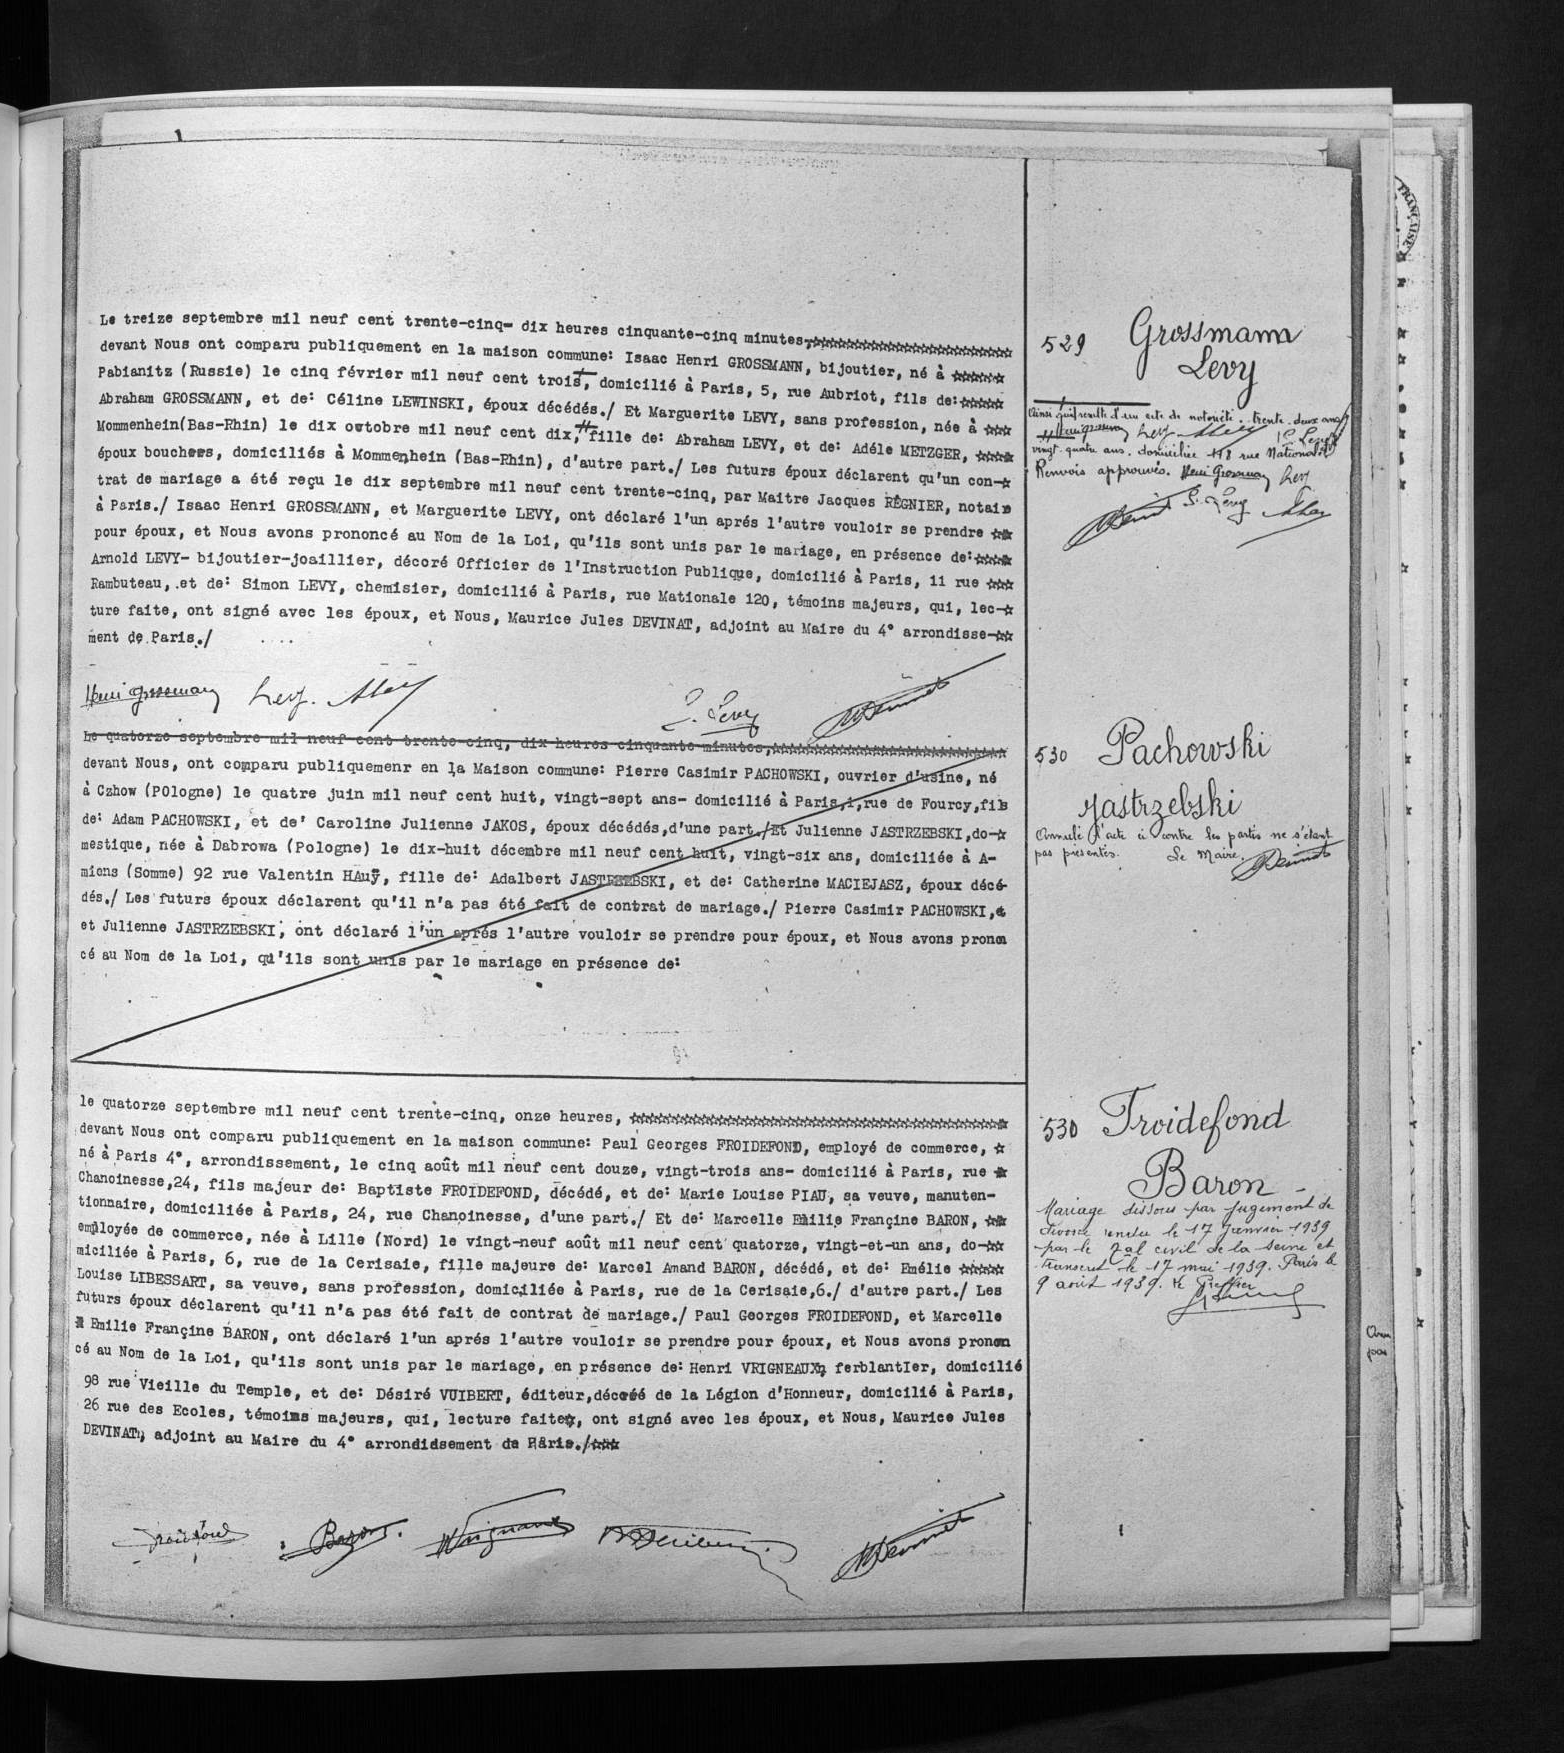Le treize septembre mil neuf cent trente-cinq-dix heures cinquante-cinq minutes, ****
devant Nous ont comparu publiquement en la maison commune: Isaac Henri GROSSMANN, bijoutier, né à ****
Pabianitz (Russie) le cinq février mil neuf cent trois, domicilié à Paris, 5, rue Aubriot, fils de: ****
Abraham GROSSMAN, et de: Céline LEWINSKI, époux décédés ./ Et Marguerité LEVY, sans profession, née à ***
Momenhein (Bas-Rhin) le dix octobre mil neuf cent dix, fille de: Abraham LEVY, et de: Adéle METZGER, ****
époux bouchees, domiciliés à Mommenhein (Bas-Rhin), d'autre part ./ Les futurs époux déclarent qu'un con -*
trat de mariage a été reçu le dix septembre mil neuf cent trente-cinq, par Maitre Jacques REGNIER, notai e
à Paris ./ Isaac Henri GROSSMAN, et Marguerite LEVY, ont déclaré l'un aprés l'autre vouloir se prendre **
pour époux, et Nous avons prononcé au Nom de la Loi, qu'ils sont unis par le mariage, en présence de: ****
Arnold LEVY-bijoutier-joaillier, décoré Officier de l'Instruction Publique, domicilié à Paris, 11 rue ***
Rambuteau, et de: Simon LEVY, chemisier, domicilié à Paris, rue Mationale 120, témoins majeurs, qui, lec -*
ture faite, ont signé avec les époux et Nous, Maurice Jules DEVINAT, adjoint au Maire du 4° arrondisse -**
ment de Paris ./
Le quatorze septembre mil neu cent trente cinq, dix heures cinquante minutes, ****
devant Nous, ont comparu publiquemenr en la Maison commune: Pierre Casimir PACHOWSKI, ouvrier d'usine, né
à Czhow (P0logne) le quatre juin mil neuf cent huit, vingt-sept ans-domicilié à Paris, 1, rue de Fourcy, fils
de: Adam PACHOWSKI, et de: Caroline Julienne JAKOS, époux décédés, d'une part ./ Et Julienne JASTRZEBSKI, do -*
mestique, née à Dabrowa (Pologne) le dix-huit décembre mil neuf cent huit, vingt-six ans, domiciliée à A-
miens (Somme) 92 rue Valentin HAuÿ, fille de: Adalbert JASTERBSKI, et de: Catherine MACIEJASZ, époux décé-
dés ./ Les futurs époux déclarent qu'il n'a pas été fait de contrat de mariage ./ Pierre Casimir PACHOWSKI,
et Julienne JASTRZEBSKI, ont déclaré l'un aprés l'autre vouloir se prendre pour époux, et Nous avons pronn
cé au nom de la Loi, qu'ils sont unis par le mariage en présence de:
le quatorze septembre mil neuf cent trente-cinq, onze heures, ****
devant Nous ont comparu publiquement en la maison commune: Paule Georges FROiDEFOND, employé de commerce, *
né à Paris 4° arrondissement, le cinq août mil neuf cent douze, vingt-trois ans-domicilié à Paris, rue *
Chanoinesse, 24, fils majeur de: Baptiste FROIDEFON, décédé, et de: Marie Louise PIAU, sa veuve, manuten-
tionnaire, domiciliée à Paris, 24, rue Chanoinesse, d'une part ./ Et de: Marcelle Amilie Françine BARON, **
employée de commerce, née à Lille (Nord) le vingt-neuf août mil neuf cent quatorze, vingt-et-un ans, do -**
miciliée à Paris, 6, rue de la Cerisaie, fille majeure de: Marcel Amand BARON, décédé, et de: Emélie ****
Louise LIBESSART, sa veuve, sans profession, domiciliée à Paris, rue de la Ceriseraie, 6 ./ d'autre part ./ Les
futurs époux déclarent qu'il n'a pas été fait de contrat de mariage ./ Paul Georges FROIDEFOND, et Marcelle
Emilie Françine BARON, ont déclaré l'un aprés l'autre vouloir se prendre pour époux, et Nous avons pronon
cé au Nom de la Loi, qu'ils sont unis par le mariage, en présence de: Henri VRIGNEAUX, ferblantIer, domicilié
98 rue Vieille du Temple, et de: Désiré VUIBERT, éditeur, décéé de la Légion d'Honneur, domicilié à Paris,
26 rue des Ecoles, témoins majeurs, qui, lecture faite *, ont signé avec les époux, et Nous, Maurice Jules
DEVINAT, adjoint au Maire du 4° arrondissement de Paris ./***
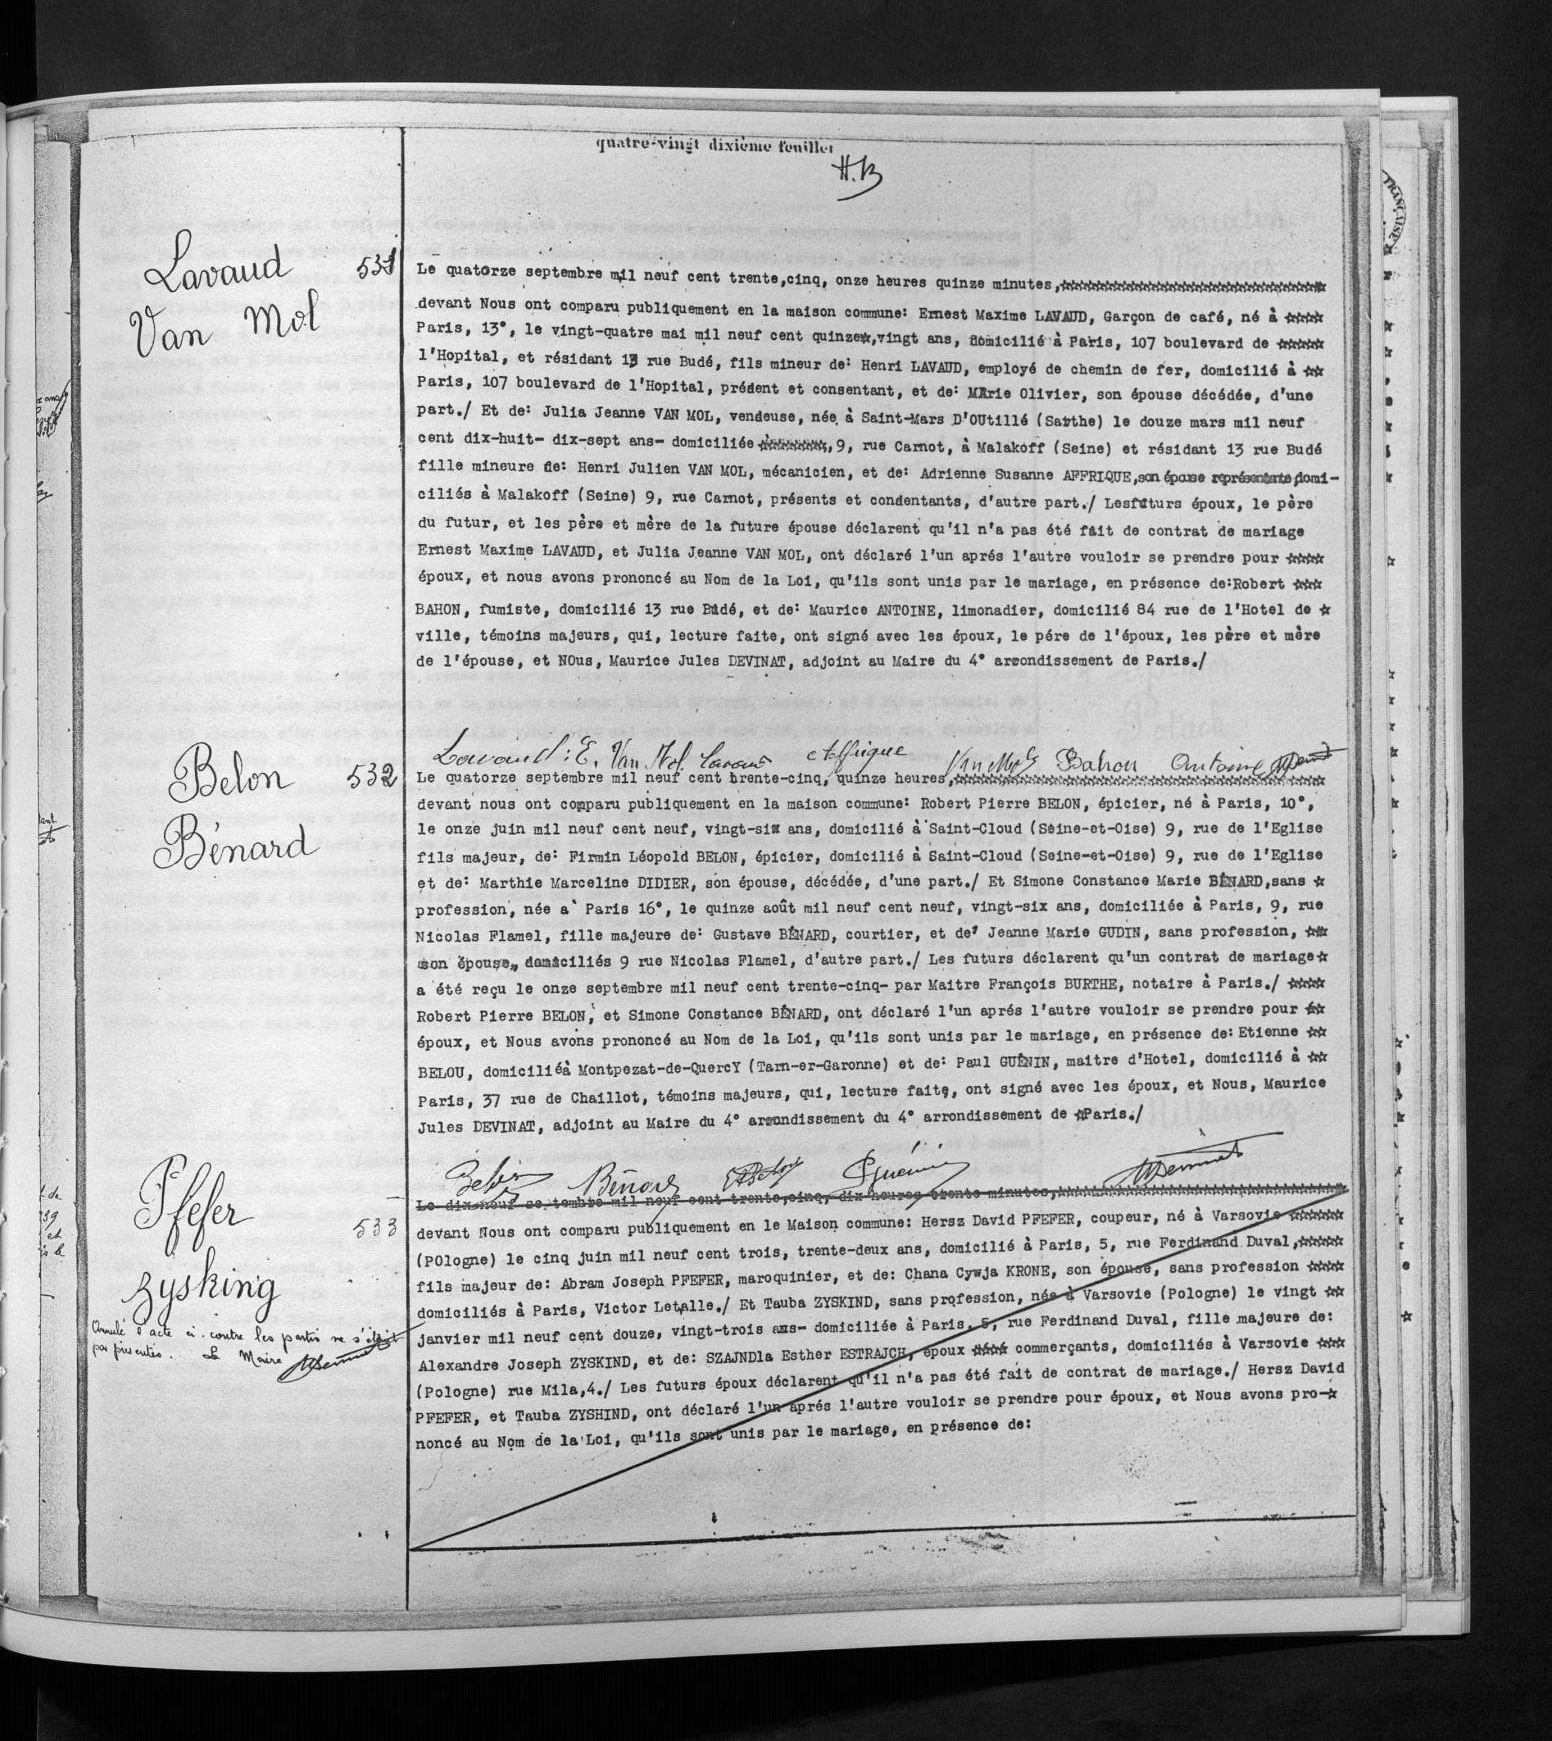Le quatorze septembre mil neuf cent trente, cinq, onze heures quinze minutes, ****
devant Nous ont comparu publiquement en la maison commune: Ernest Maxime LAVAUD, Garçon de café, né à ***
Paris, 13°, le vingt-quatre mai mil neuf cent quinze *, vingt ans, domicilié à Paris, 107 boulevard de ****
l'Hopital, et résidant 13 rue Budé, fils mineur de: Henri LAVAUD, employé de chemin de fer, domicilié à **
Paris, 107 boulevard de l'Hopital, présent et consentant, et de: MArie Olivier, son épouse décédée, d'un
part ./ Et de: Julia Jeanne VAN MOL, vendeuse, née à Saint-Mars D'OUtillé (Sarthe) le douze mars mil neuf
cent dix-huit dix-sept ans-domiciliée ****, 9, rue Carnot, à Malakoff (Seine) et résidant 13 rue Budé
fille mineure de: Henri Julien VAN MOL, mécanicien, et de: Adrienne Suzanne AFFRIQUE, son épouse représentante domi-
ciliés à Malakoff (Seine) 9, rue Carnot, présents et condentants, d'autre part ./ Les futurs époux, le père
du futur, et les père et mère de la future épouse déclarent qu'il n'a pas été fait de contrat de mariage
Ernest Maxime LAVAUD, et Julia Jeanne VAN MOL, ont déclaré l'un aprés l'autre vouloir se prendre pour ****
époux, et nous avons prononcé au Nom de la Loi, qu'ils sont unis par le mariage, en présence de Robert ****
BAHON, fumiste, domicilié 13 rue Bidé, et de: Maurice ANTOINE, limonadier, domicilié 84 rue de l'Hotel de *
ville, témoins majeurs, qui, lecture faite, ont signé avec les époux, le pére de l'époux, les père et mère
de l'épouse, et N0us, Maurice Jules DEVINAT, adjoint au Maire du 4° arrondissement de Paris ./
Le quatorze septembre mil neuf cent brente-cinq, quinze heures, ****
devant nous ont conparu publiquement en la maison commune: Robert Pierre BELON, épicier, né à Paris, 10°,
le onze huin mil neuf cent neuf, vingt-six ans, domicilié à Saint-Cloud (Seine-et-Oise) 9, rue de l'Eglise
fils majeur, de: Firmin Léopold BELON, épicier, domicilié à Saint-Cloud (Seine-et-Oise) 9, rue de l'Eglise
et de: Marthie Marceline DIDIER, son épouse, décédée, d'une part ./ ET Simone Constance Marie BENARD, sans*
profession, née à Paris 16°, le quinze août mil neuf cent neuf, vingt-six ans, domiciliée à Paris, 9, rue
Nicolas Flamel, fille majeure de: Gustave BENARD, courtier, et de Jeanne Marie GUDIN, sans profession ,**
son épouse, domiciliés 9 rue Nicolas Flamel, d'autre part ./ Les futurs déclarent qu'un contrat de mariage *
a été reçu le onze septembre mil neuf cent trente-cinq-par Maitre François BURTHE, notaire à Paris ./****
Robert Pierre BELON, et Simone Constance BENARD, ont déclaré l'un aprés l'autre vouloir se prendre pour **
époux, et Nous avons prononcé au Nom de la Loi, qu'ils sont unis par le mariage, en présence de: Etienne **
BELOU, domicilié à Montpezat-de-QuercY (Tarn-er-Garonneà et de: Paul GUENIN, maitre d'Hotel, domicilié à **
Paris, 37 rue de Chaillot, témoins majeurs, qui, lecture faite, ont signé avec les époux, et Nous, Maurice
Jules DEVINAT, adjoint au Maire du 4° arrondissement du 4° arrondissement de * Paris ./
Le dix neuf septembre mil neuf cent trente,cinq, dix heures trente minutes, ****
devant Nous ont comparu publiquement en la Maison commune: Hersz David PFEFER, coupeur, né à Varsovie ****
(POlogne) le cinq juin mil neuf cent trois, trente-deux ans, domicilié à Paris, 5, rue Ferdinand Duval, ****
fils majeur de: Abram Joseph PREFER, maroquinier, et de: Chana Cywja KRONE, son épouse, sans profession ****
domiciliés à Paris, Victor Letalle ./ Et Tauba ZYSKIND, sans profession, née à Varsovie (Pologne) le vingt **
janvier mil neuf cent douze, vingt-trois ans-domiciliée à Paris, 5, rue Ferdinand Duval, fille majeure de:
Alexandre Joseph ZYSKIND, et de: SZAKNDla Esther ESTRAJCH, époux *** commerçants, domiciliés à Varsovie ***
(Pologne) rue Mila, 4 ./ Les futurs époux déclarent qu'il n'a pas été fait de contrat de mariage ./ Hersz David
PFEFER, et Tauba ZYSHIND, ont déclaré l'un aprés l'autre vouloir se prendre pour époux, et Nous avons pro -*
noncé au Nom de la Loi, qu'ils sont unis par le mariage, en présence de: